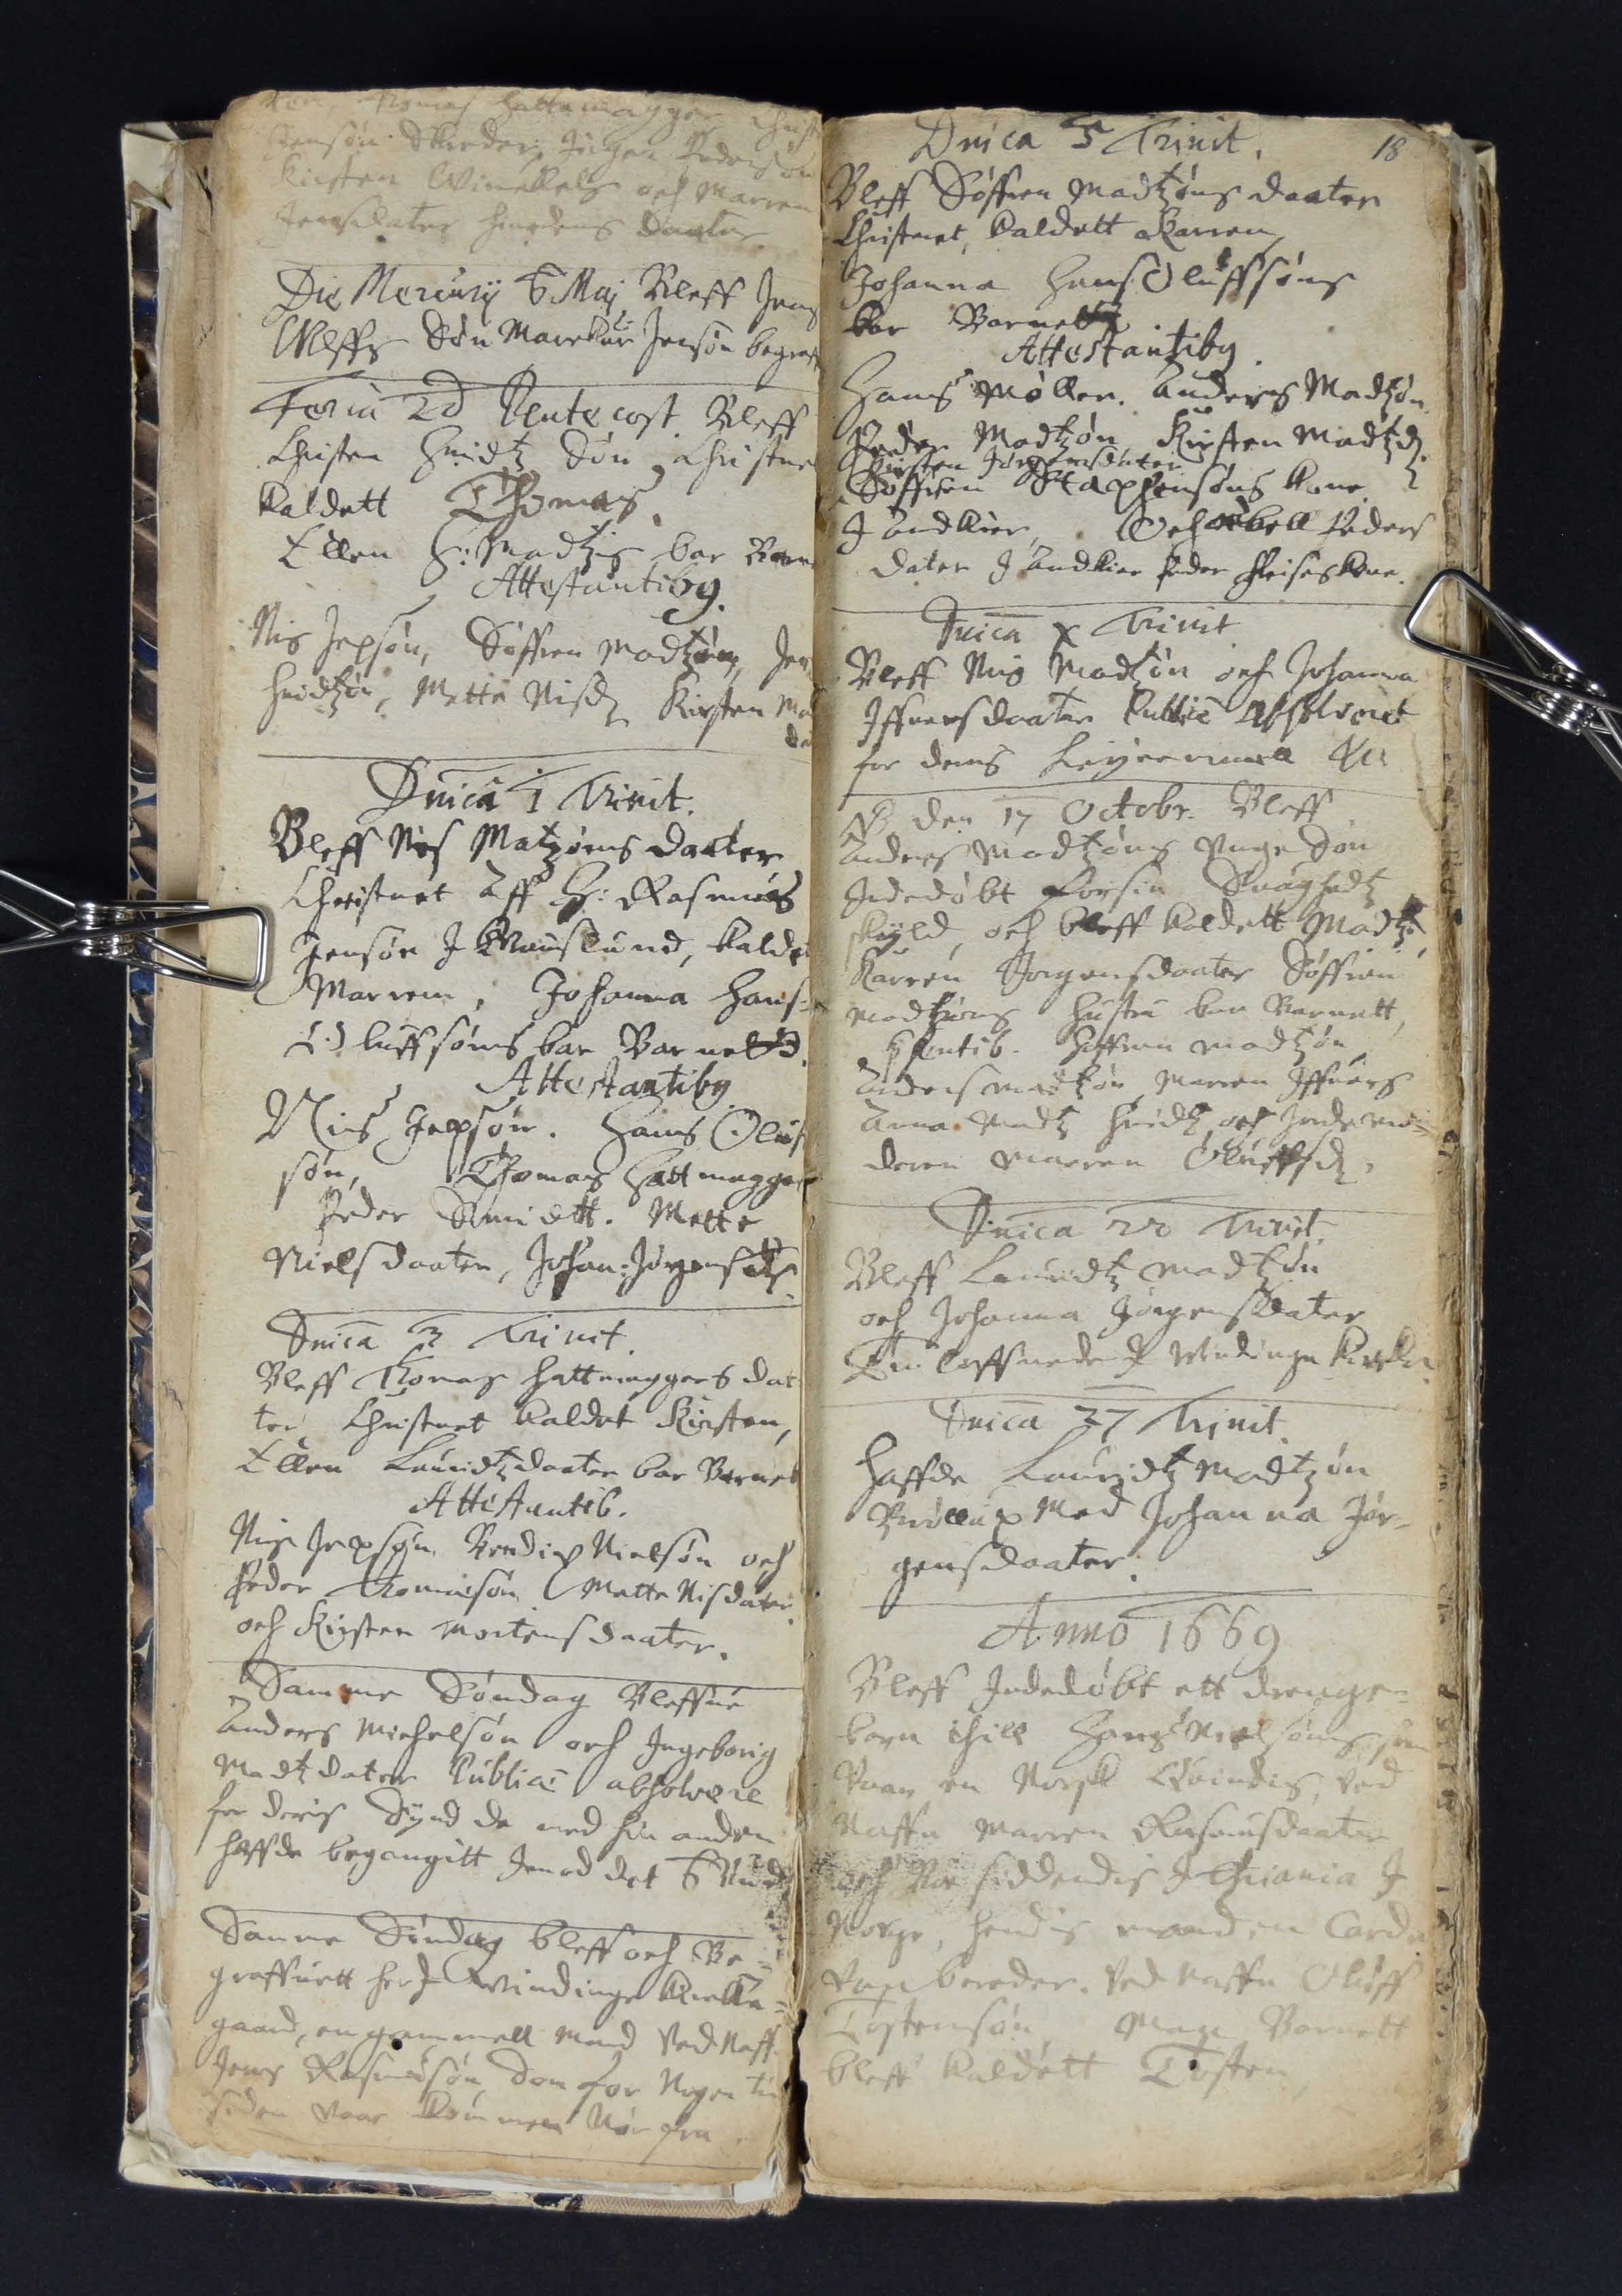och Thomas Hattemagger
Jensøn Skreder, Jørgen Pedersøn
Kirsten Wienckels och Marren
Jensdater hiendes Daater.
Die Mercurij. 6 Maj. Bleff Jens
Vlffs Søn Marckus Jensøn begraf 
Feria 2 d Pentecost. Bleff
Christen Hvidtz Søn Christne 
kaldett Thomas.
Ellen H: Madtzis bar Barn 
Attestantiby.
Nis Jepsøn, Søffren Madtzøn, Jen 
Hvidtzøn. Mette Nisd. Kirsten Ma 
da 
Dnica 1 Trinit.
Bleff Nis Madtzøns Daater
Christnet Aff H: Rasmus
Jensøn I Gauerslund, kaldet 
Marren, Johanna Hans
Oluffsøns bar Barnet
Attestantiby
Nis Jepsøn. Hans Oluf 
søn, Thomas Hattemagger
Peder Smidtt. Mette
Nielsdaater, Johan:
Dnica 3 Trinit
Bleff Thomas Hattemaggers Dat
ter Christnet kaldet Kirsten
Ellen Lauridtzdaater bar Barnet
Attestantib.
Nis Jepsøn. Bendix Nielsøn och
Peder Thomasøn. Mette Nisdater
och Kirsten Mortensdaater.
Samme Søndag. Bleffue
Anders Michelsøn och Ingeborig 
Madtzdater Publica absolvere
for deris Synd de med hin anden
haffde begangitt Imod det 6 Bud
Samme Søndag bleff och Be¬
graffuett her I Windinge Kirke¬
gaard, en gammel Mand ved Naff.
Jens Rasmussøn som for nogen tid 
siden vaar kommen nør fra
18
Dnica 5 Trinit.
Bleff Søffren Madtzøns Daater
Christnet, kaldett Karren
Johanna Hans Oluffsøns
bar Barnett
Attestantiby
Hans Møller. Anders Madtzøn.
Peder Madtzøn Kirsten Madtzd.
Kirsten Jørgensdater.
Søffren Staphansøns Kone
I Andkier, och ##bell Peders
dater I Andkier Peder Frises Kone
Dnica X Trinit
Bleff Nis Madtzøn och Johanna
Iffuersdaater Public absolveret
for deres leyermaall
NB. den 17 Octobr. Bleff
Anders Madtzøns Suge Søn
Indedøbt for sin Suaghedtz
skyld, och bleff kaldett Madtz.
Karren Jorgensdaater Søffren
Madtzøns Hustru bar Barnett
Søffren Madtzøn
Anders Madtzøn. Marren Iffuers
Anna Madtz Hvidtz, och Jordemo
deren Marren Oluffsd
Dnica 20 Trinit
Bleff Lauridtz Madtzøn
och Johanna Jørgensdater
Troloffuede I Windinge Kircke
Dnica 27 Trinit
Haffde Lauridtz Madtzøn
Brøllup med Johanna Jør¬
gensdaater
Anno 1669
Bleff Indedøbt ett drenge¬
barn thill Hans Nielsøns som
vaar en Norsk Qvindis, ved
Naffn Marren Rasmusdaater
och Boe siddendis I Christiania I
Norge, hendis mand en Cardu 
Torstensøn. Men Barnett
bereder. ved naffn Oluff
bleff kaldett Torsten
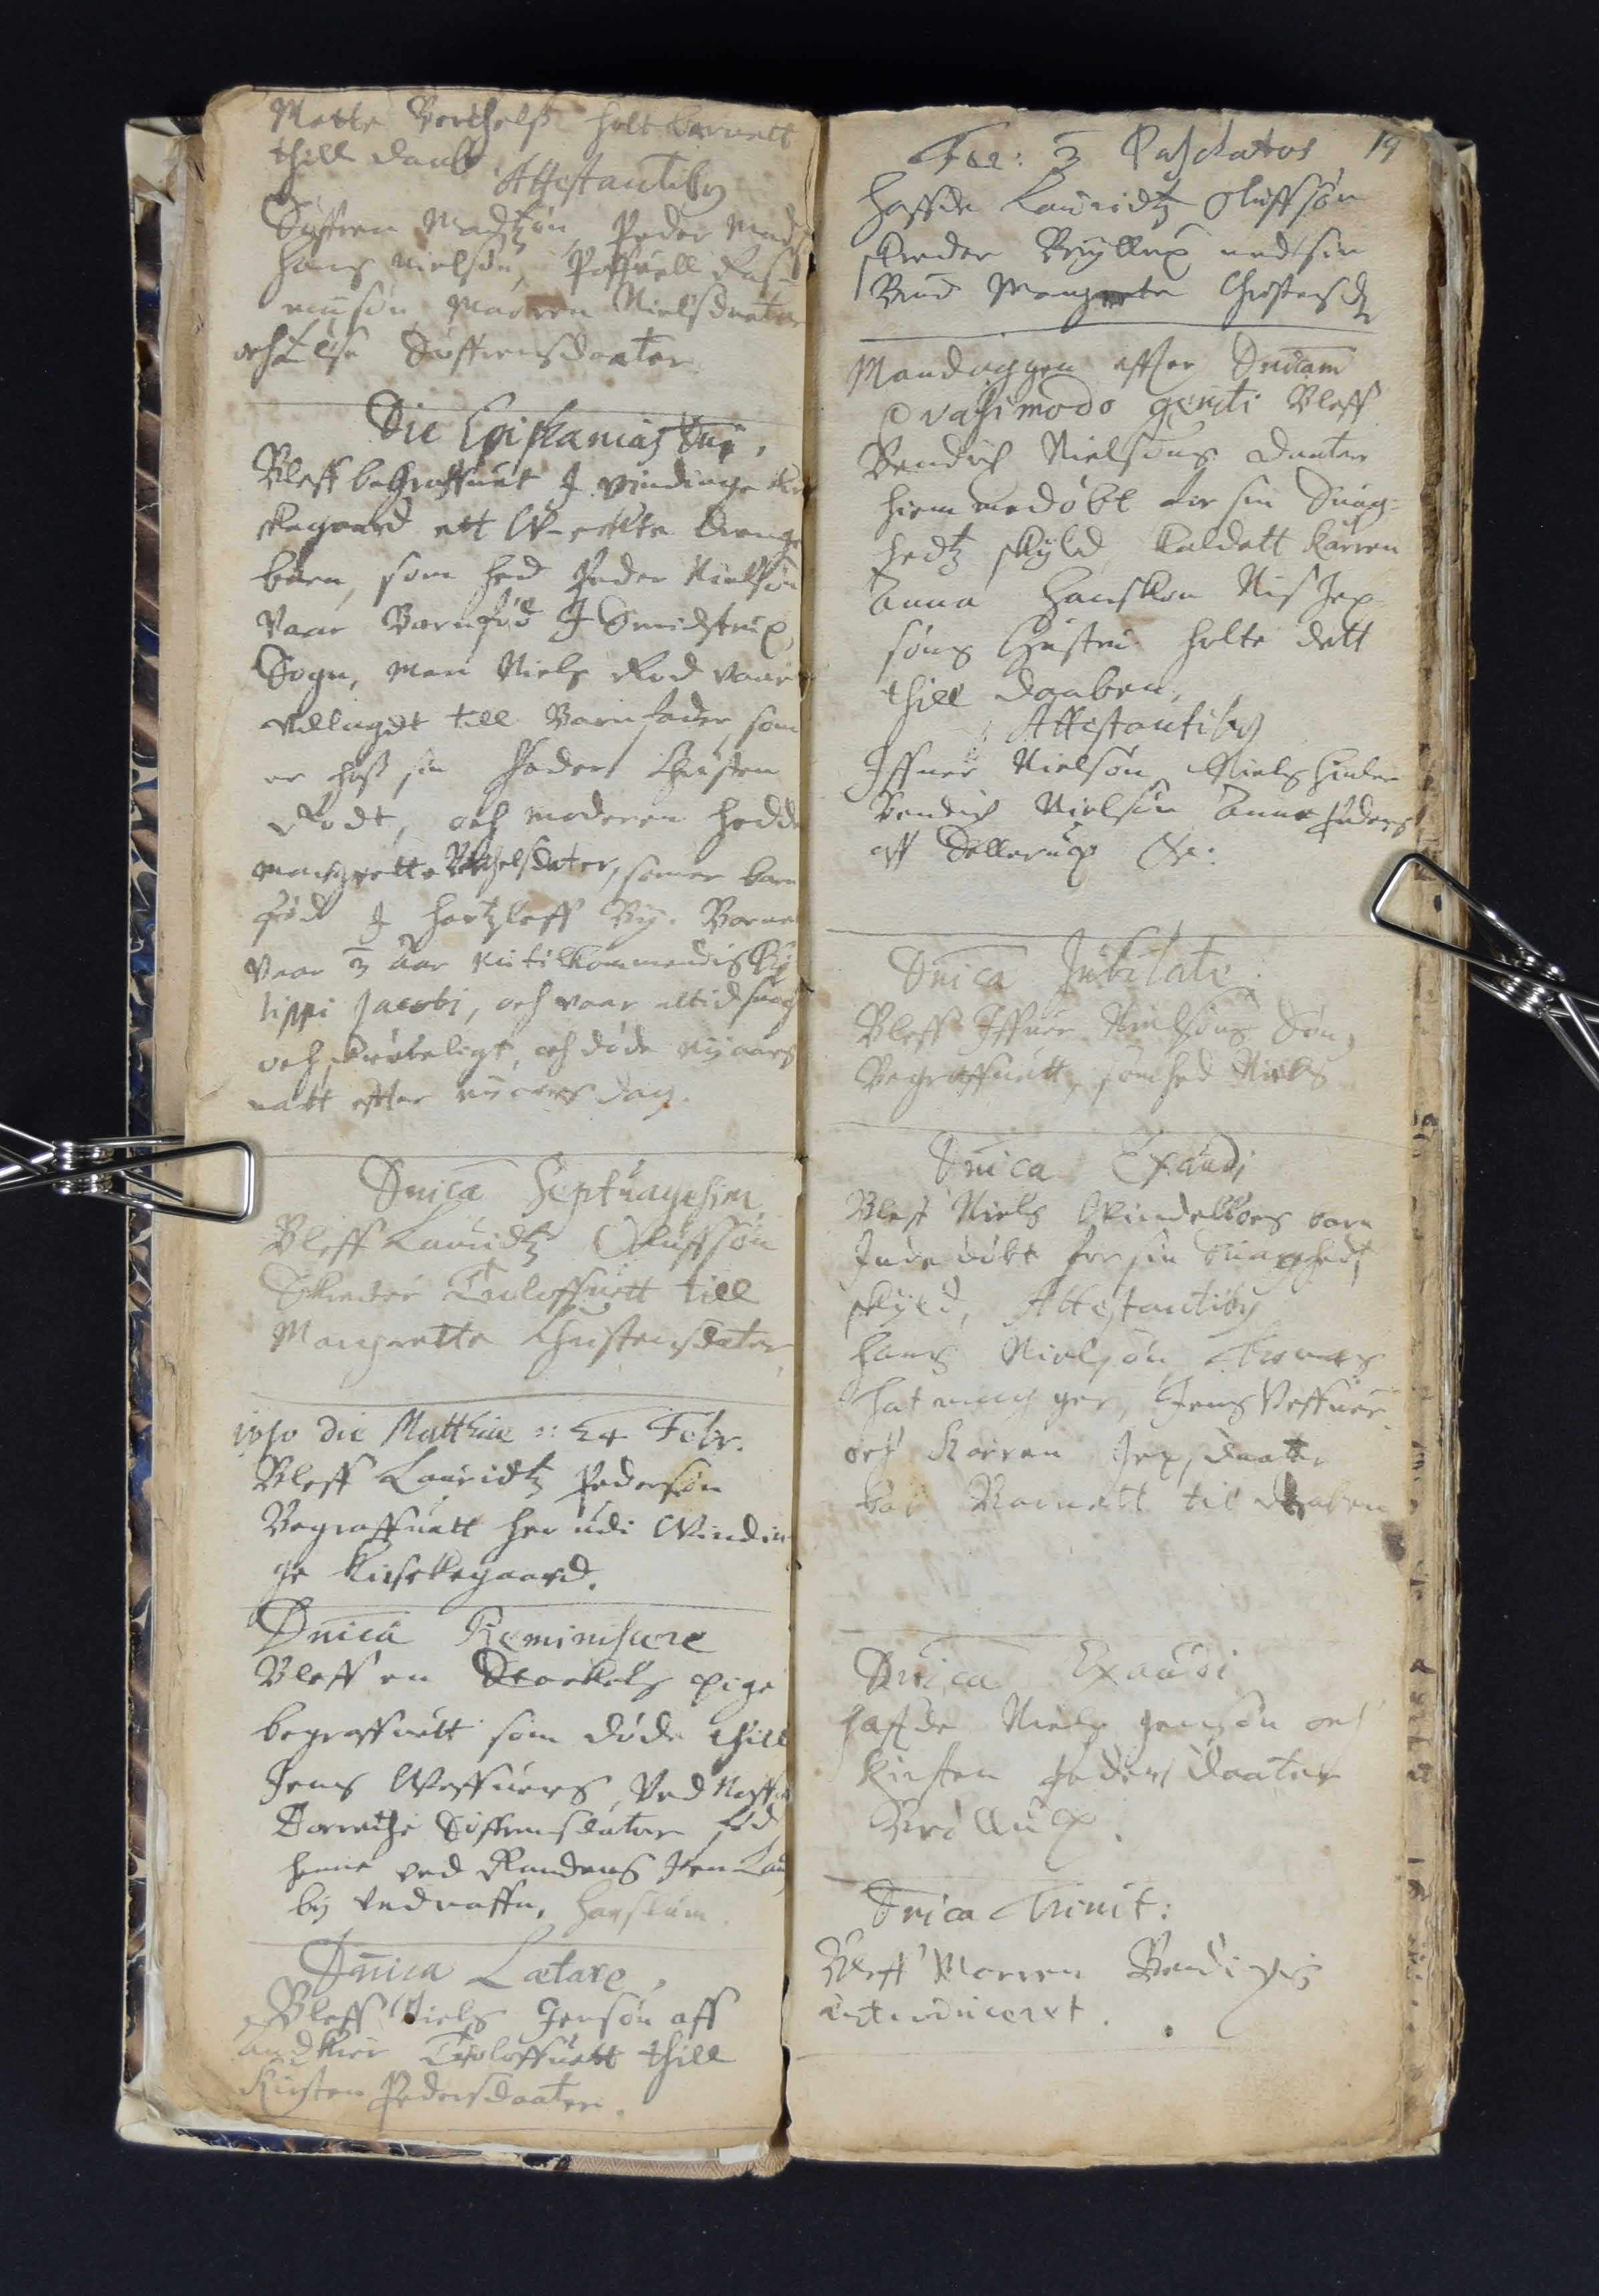Mette Berthelss holt barnett
thill daab
Attestantiby
Søffren Madtzøn. Peder Madtz 
Hans Nielsøn, Poffuell Ras¬
musøn. Marren Nielsdaater
och Else Søffrensdaater
Die Epiphanias
Bleff begraffuet I Vindinge Kir
ckegaard ett V-eckte Drenge
barn, som hed Peder Nielsøn.
Vaar Barnefød I Smidstrup
Sogn, Men Niels Rod vaar
vdlagdt till Barnefader som
er hoss sin Fader Christen
Rodt, och moderen hedde
Margrette Michelsdater, som er barn
fød I Hertzløff By. Barne 
vaar 3 aar nu tilkommendis Pi
lippi Jacobi, och vaar altid suag 
och skrøbeligt, och døde Nyaars
natt efter nyaars dag.
Dnica Septuagesim
Bleff Lauridtz Oluffsøn
Skreder Troloffuett till
Margrette Christensdater 
i## die Matthiae## ##: 24 Febr.
Bleff Lauridtz Pedersøn
Begraffuett her udi Windin¬
ge Kirkegaard.
Dnica Reminescere.
Bleff en Stackels pige
begraffuett som døde thill
Jens Weffuers, Ved naffn
Dorethe Søffrensdater, fød 
henne ved Randers I en Lands
by ved naffn
Dnica Latare
Bleff Niels Jensøn aff
Andkier Troloffuett thill 
Kirsten Pedersdaater
19
Fer 3 Paschatos
Haffde Lauridtz Oluffsøn 
skreder Bryllup med sin
Brud Margrete Christensd
Mandaggen effter Dnicam
Qvasimodo geniti Bleff
Bendix Nielsøns Daater
hiem medøbt for sin Suag¬
hedtz skyld kaldett Karren
Anna Hans kon Nis Jep¬
søns Hustru holte dett
thill daaben
Attestantiby
Iffuer Nielsøn Niels Hiuler.
Bendix Nielsøn Anna Peders
aff Sellerup
Dnica Jubilate
Bleff Iffuer Nielsøns Søn
Begraffuett som hed Niels
Dnica Exaudi
Bleff Niels Windelboes barn
Inde døbt for sin Suaghedtz
skyld, Attestantiby
Hans Nielsøn Thomas
Hatmagger, Jens Veffuer
och Karren Jepsdaatter
bar Barnett til Daaben.
Dnica Exaudi
haffde Niels Jensøn och
Kirsten Pedersdaater
Brøllup.
Dnica Trinit:
Bleff Marren Bendixis
introduceret